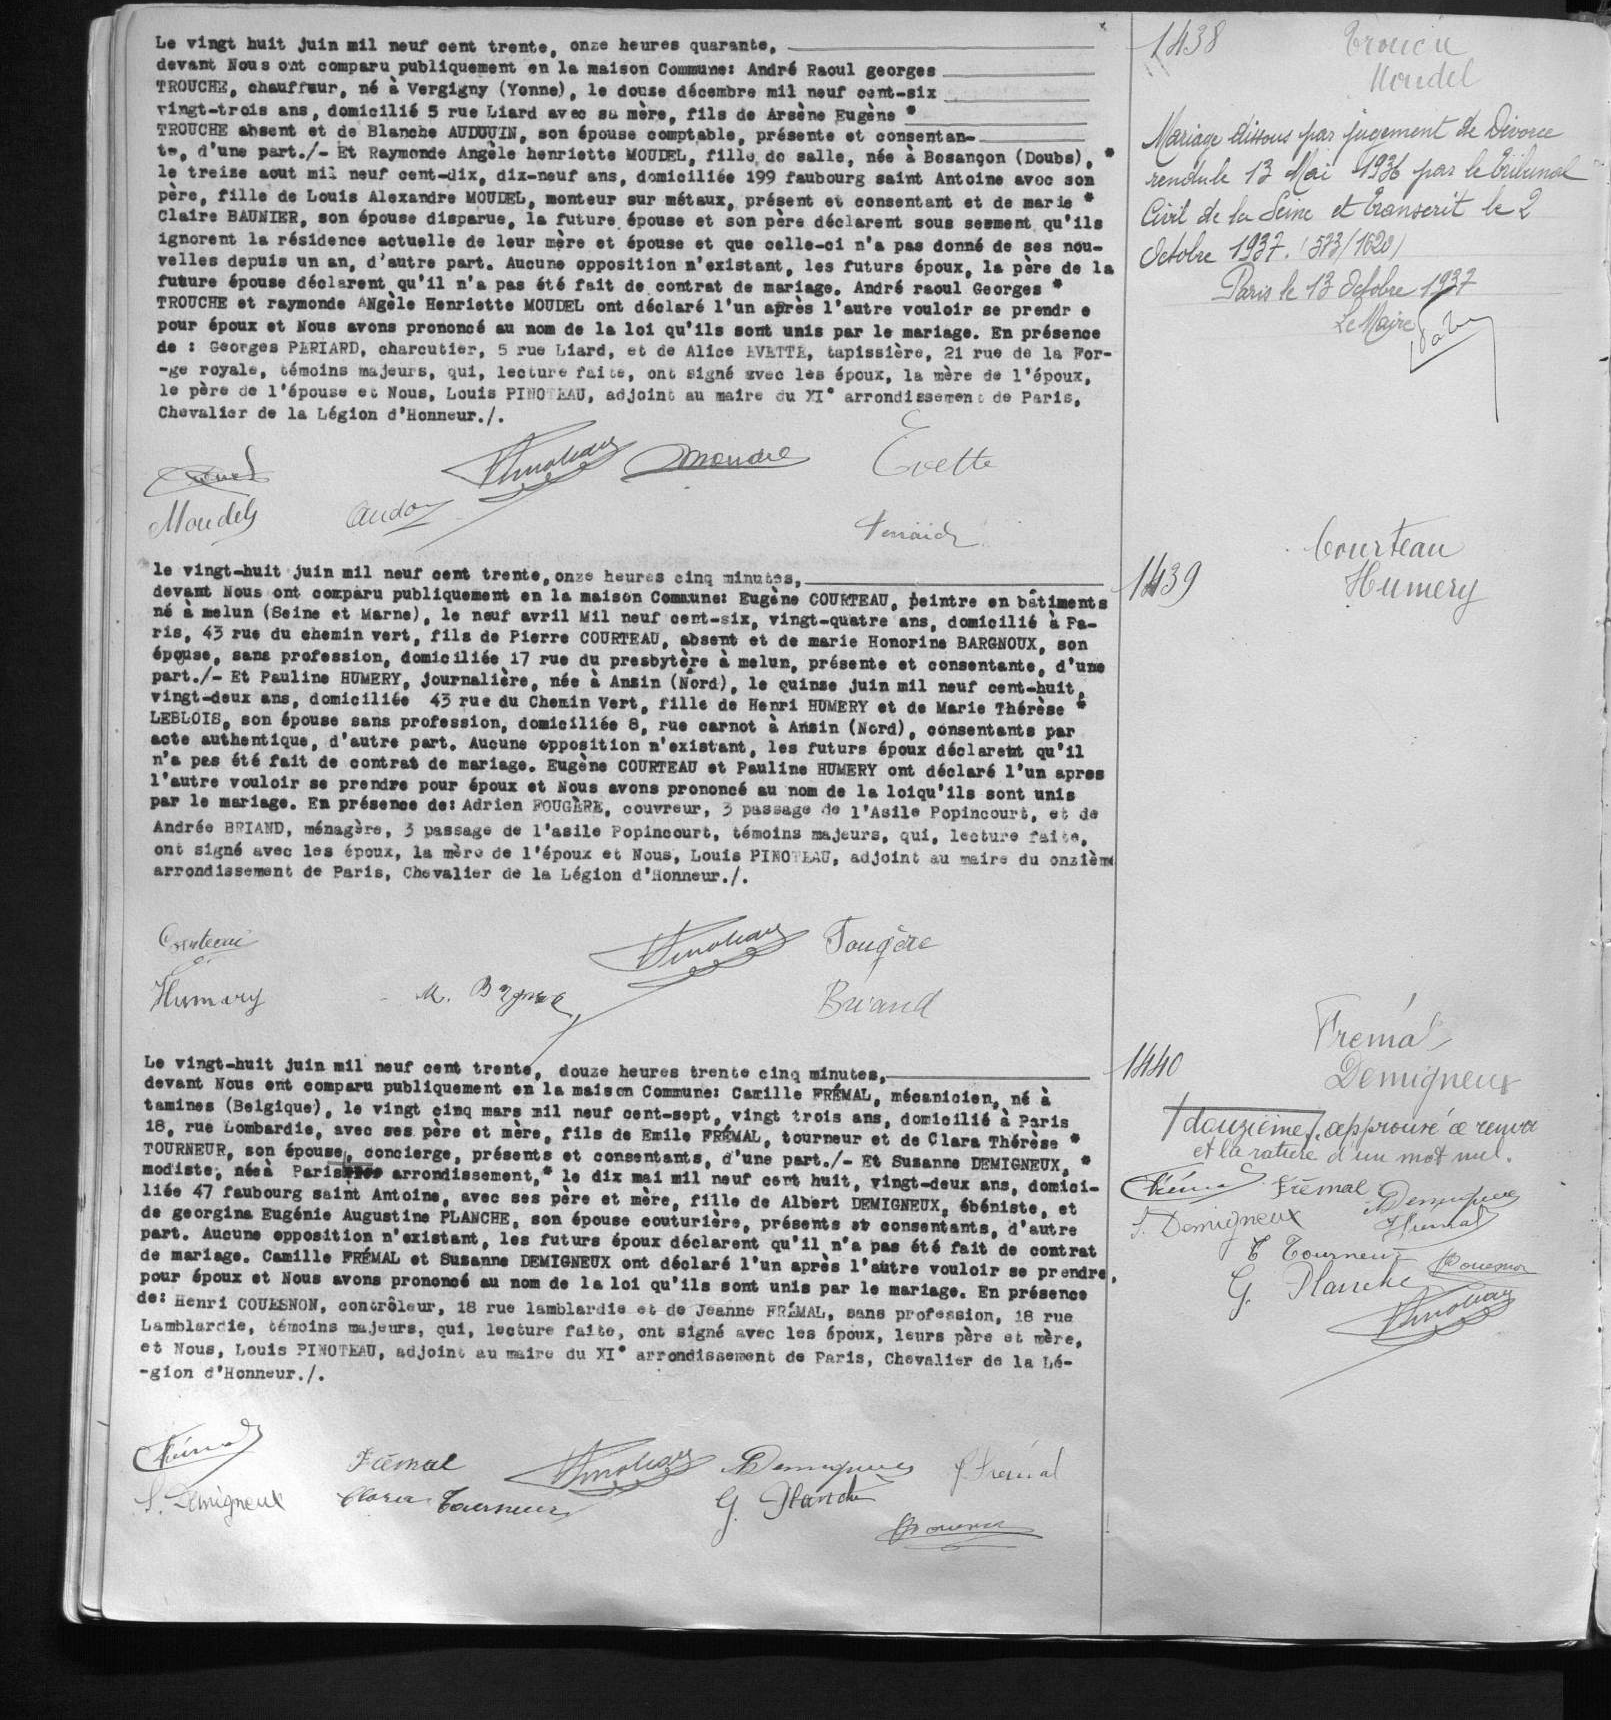Le vingt huit juin mil neuf cent trente, onze heures quarante, -
devant Nous ont comparu publiquement en la maison Commune André Raoul georges -
TROUCHE, chauffeur, né à Vergigny (Yonne), le douze décembre mil neuf cent-six -
vingt-trois ans, domicilié 5 rue Liard avec a mère, fils de Arsène Eugène *-
TROUCH absent et de Blanch ADUUIN, son épouse comptable, présente et consentan -
te, d'une part ./-Et Raymonde Angèle henriette MOUDEL, fille do salle, née à Besançon (Doubs) ,*
le treize aout mil neuf cent-dix, dix-neuf ans, domiciliée 199 faubourg saint Antoine avec son
père, fille de Louis Alexandre MOUDEL, monteur sur métaux, présent et consentant et de marie *
Claire BAUNIER, son épouse disparue, la future épouse et son père déclarent sous serment qu'ils
ignorent la résidence actuelle de leur mère et épouse et que celle-ci n'a pas donné de ses nou-
velles depuis un an, d'autre part. Aucune opposition n'existant, les futurs époux, la père de la
future épouse déclarent qu'il n'a pas été fait de contrat de mariage. André raoul Georges *
TROUCHE et raymonde Angèle Henriette MOUDEL ont déclaré l'un après l'autre vouloir se prendr e
pour époux et Nous avons prononcé au nom de la loi qu'ils sont unis par le mariage. En présence
de: Georges PARIARD, charcutier, 5 rue Liard, et de Alice EVETTE, tapissière, 21 rue de la For-
ge royale, témoins majeurs, qui, lecture faite, ont signé avec les époux, la mère de l'époux,
le père de l'épouse et Nous, Louis PINOTEAU, adjoint au maire du XI ° arrondissemen de Paris,
Chevalier de la légion d'Honneur ./.
le vingt-huit juin mil neuf cent trente, onze heures cinq minutes, -
devant Nous ont comparu publiquement en la maison Commune: Eugène COURTEAU, peintre en bâtiments
né à melun (Seine et Marne), le neuf avril Mil neuf cent-six, vingt-quatre ans, domicilié à Fa-
ris, 43 rue du chemin vert, fils de Pierre COURTEAU, absent et de marie Honorine BARGNOUX, son
épouse, sans profession, domiciliée 17 rue du presbytère à melun, présente et consentante, d'une
part ./-Et Pauline HUMERY, journalière, née à Anzin (Nord), le quinze juin mil neuf cent-huit,
vingt-deux ans, domiciliée 43 rue du Chemin Vert, fille de Henri HUMERY et de Marie Thérèse *
LEBLOIS, son épouse sans profession, domiciliée 8, rue carnot à Ansin (Nord), consentants par
acte authentique, d'autre part. Aucune opposition n'existant, les futurs époux déclarent qu'il
n'a pas été fait de contrat de mariage. Eugène COURTEAU et Pauline HUMERY ont déclaré l'un apres
l'autre vouloir se prendre pour époux et Nous avons prononcé au nom de la loi qu'ils sont unis
par le mariage. En présence de: Adrien FOUGERE, couvreur, 3 passage de l'Asile Popincourt, et de
Andrée BRIAND, ménagère, 3 passage de l'asile Popincourt, témoins majeurs, qui, lecture faite,
ont signé avec les époux, la mère de l'époux et Nous, Louis PINOTEAU, adjoint au maire du onzième
arrondissement de Paris, Chevalier de la Légion d'Honneur ./.
Le vingt-huit juin mil neuf cent trente, douze heures trente cinq minutes -
devant Nous ont comparu publiquement en la maison Commune: Camille FREMAL, mécanicien, né à
tamines (Belgique), le vingt cinq mars mil neuf cent-sept, vingt trois ans, domicilié à Paris
18, rue Lombardie, avec ses père et mère, fils de Emile FREMAL, tourneur et de Clara Thérèse *
TOURNEUR, son épouse, concierge, présents et consentants, d'une part ./-Et Suzanne DEMIGNEUX ,*
modite, née à Paris arrondissement, * le dix mai mil neuf cent huit, vingt-deux ans, domici-
liée 47 faubourg saint Antoine, avec ses père et mère, fille de Albert DEMIGNEUX, ébéniste, et
de georgina Eugénie Augustine PLANCHE, son épouse couturière, présents et consentants, d'autre
part. Aucune opposition n'existant, les futurs époux déclarent qu'il n'a pas été fait de contrat
de mariage. Camille FREMAL et Suzanne DEMIGNEUX ont déclaré l'un après l'autre vouloir se prendre
pour époux et Nous avons prononcé au nom de la loi qu'ils sont unis par le mariage. En présence
de: Henri COUESNON, contrôleur, 18 rue lamblardie et de Jeanne FREMAL, sans profession, 18 rue
Lamblardie, témoins majeurs, qui, lecture faite, ont signé avec les époux, leurs père et mère,
et Nous, Louis PINOTEAU, adjoint au maire du XI ° arrondissement de Paris, Chevalier de la Lé-
gion d'Honneur ./.
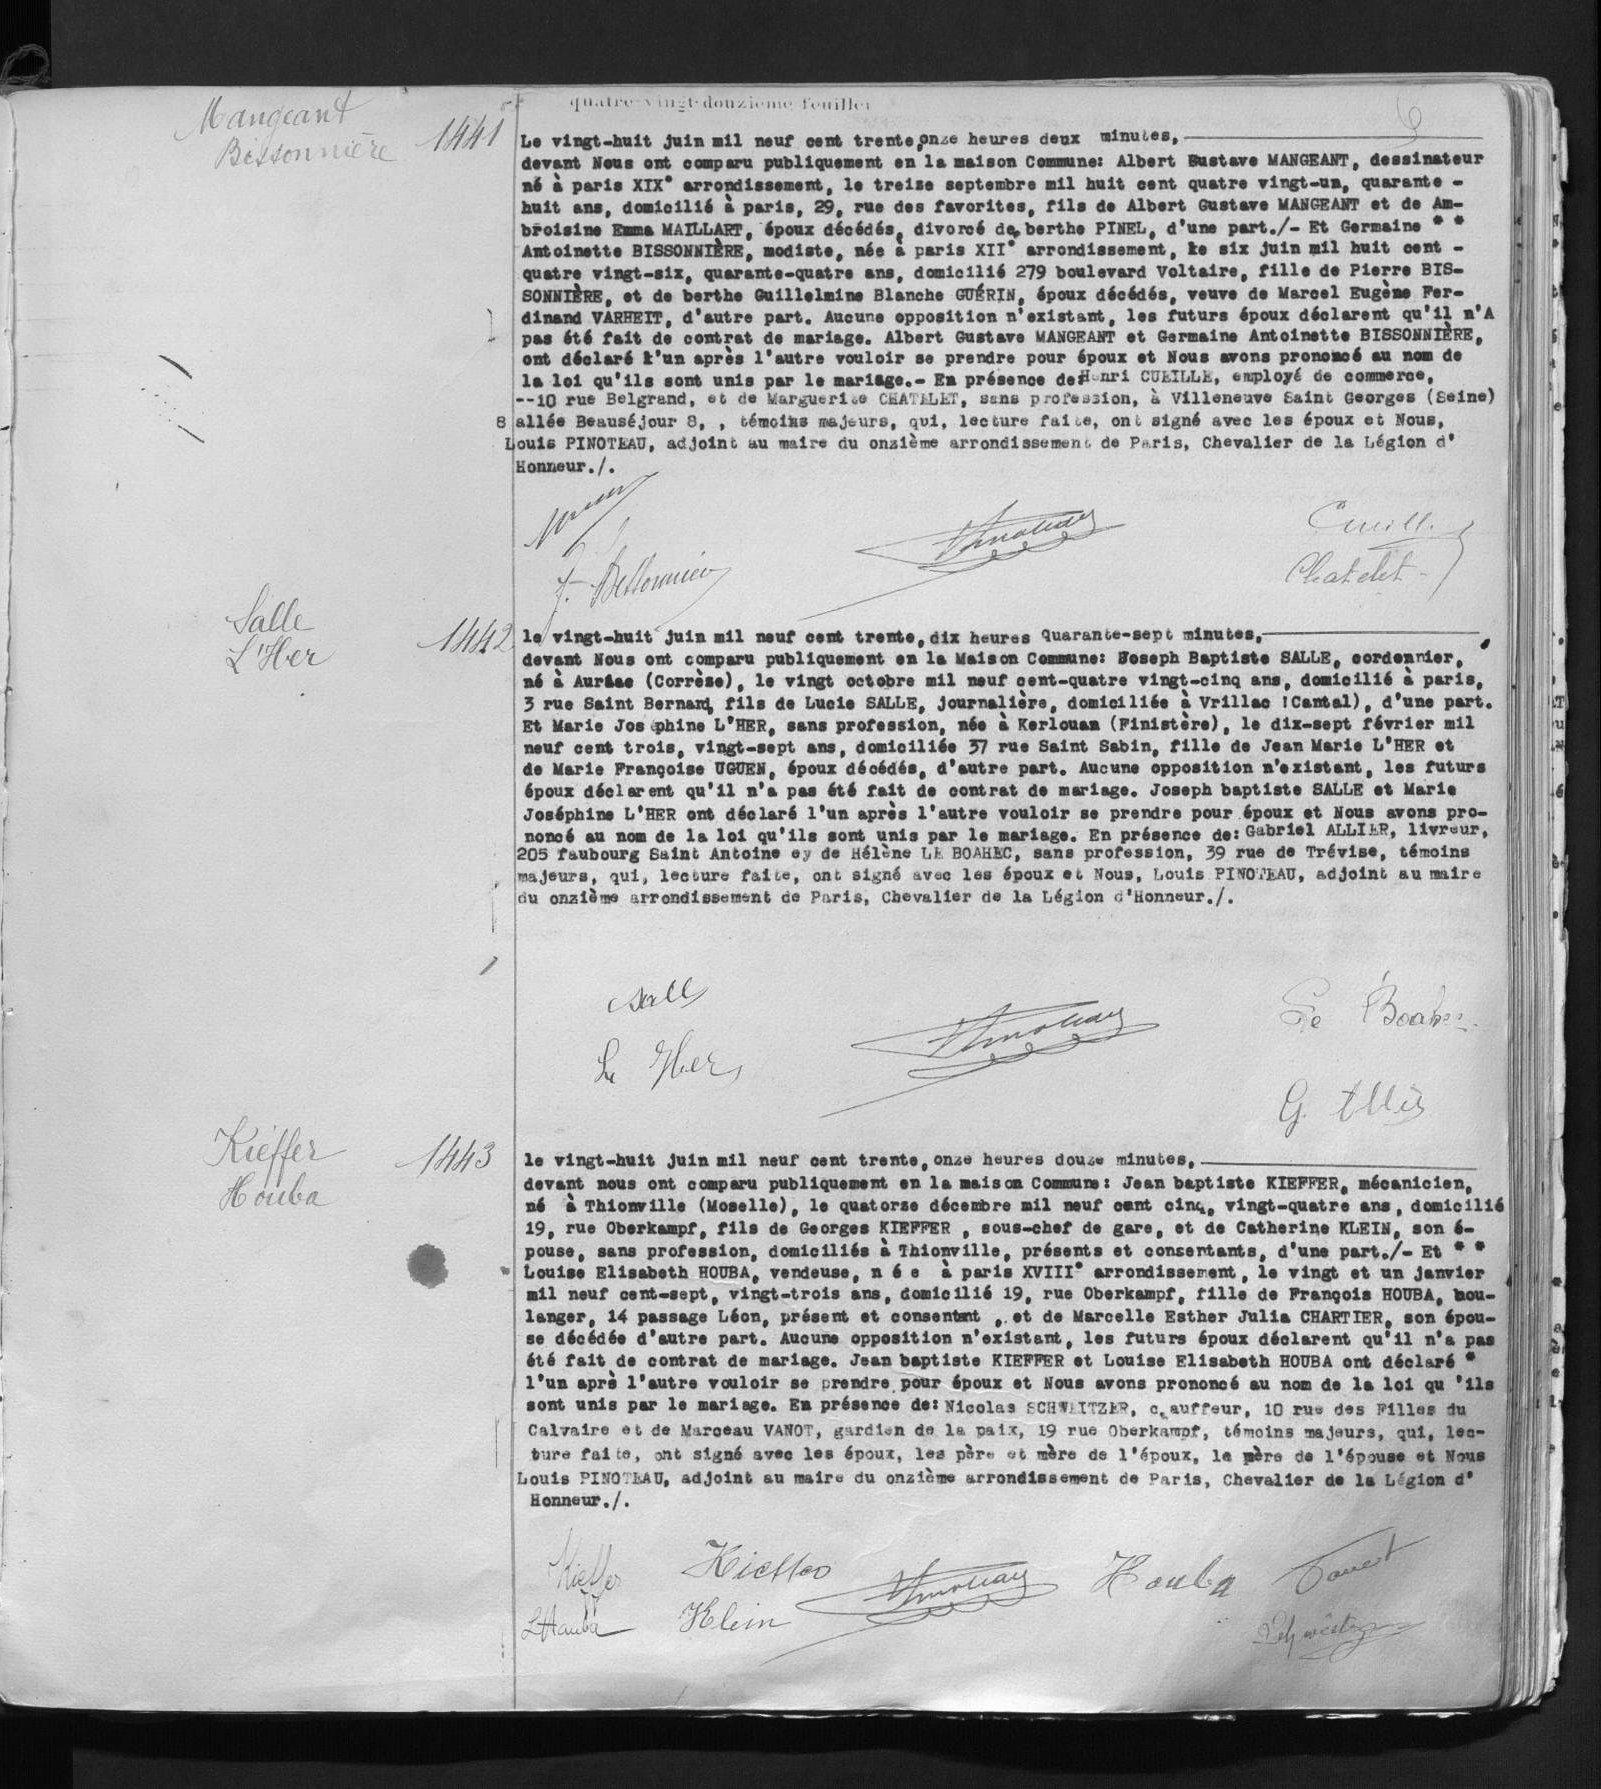Le vingt-huit juin mil neuf cent trente, onze heures deux minutes, -
devant Nous ont comparu publiquement en la maison Commune: Albert Eustave MANDEANT, dessinateur
né à paris XIX ° arrondissement, le treize septembre mil huit cent quatre vingt-un, quarante-
huit ans, domicilié à paris, 29, rue des favorites, fils de Albert Gustave MANGEANT et de Am-
broisine Emma MAILLART, époux décédés, divorcé de berthe PINEL, d'une part ./-Et Germaine **
Antoinette BISSONNIERE, modiste, née à paris XII ° arrondissement, le six juin mil huit cent-
quatre vingt-six, quarante-quatre ans, domicilié 279 boulevard Voltaire, fille de Pierre BIS-
SONNIERE, et de berthe Guillelmine Blanche GUERIN, époux décédés, veuve de Marcel Eugène Fer-
dinand VARHEIT, d'autre part. Aucune opposition n'existant, le futurs époux déclarent qu'il n'A
pas été fait de contrat de mariage. Albert Gustave MANGEANT et Germaine Antoinette BISSONIERE,
ont déclaré l'un après l'uatre vouloir se prendre pour époux et Nous avons prononcé au nom de
la loi u'ils sont unis par le mariage .-Et présence de Henri CUEILLE, employé de commerce,
-10 rue Belgrand, et de Marguerite CHATELET, sans profession, à Villeneuve Saint Georges (Seins)
allée Beauséjour 8, témoins majeurs, qui, lecture faite, ont signé avec les époux et Nous,
Louis PINOTEAU, adjoint au maire du onzième arrondissement de Paris, Chevalier de la Légion d'
Honneur ./.
Le vingt-huit juin mil neuf cent trente, dix heures quarante-sept minutes, -,
devant Nous ont comparu publiquement en la Maison Commune: Joseph Baptiste SALLE, cordonnier,
né à Aurâce (Corrèze), le vingt octobre mil neuf cent-quatre vingt-cinq ans, domicilié à paris,
3 rue Saint Bernard, fils de Lucie SALLE, journalière, domiciliée à Vrillac: Cantal), d'une part.
Et Marie Joséphine L'HER, sans profession, née à Kerlouan (Finistère), le dix-sept février mil
neuf cent trois, vingt-sept ans, domiciliée 37 rue Saint Sabin, fille de Jean Marie L'HER et
de Marie Françoise UGUEN, époux décédés, d'autre part. Aucune opposition n'existant, es futurs
époux déclarent qu'il n'a pas été fait de contrat de mariage. Joseph baptiste SALLE et Marie
Joséphine L'HER ont déclaré l'un après l'autre vouloir se prendre pour époux et Nous avons pro-
noncé au nom de la loi qu'ils sont unis par le mariage. En présence de: Gabriel ALLIER, livreur,
205 faubourg Saint Antoine ey de Hélène LE BOAHEC, sans profession, 39 rue de Trévise, témoins
majeurs, qui, lecture faite, ont signé avec les époux et Nous, Louis PINOTEAU, adjoint au maire
du onzième arrondissement de Paris, Chevalier de la Légion d'Honneur ./.
Le vingt-huit juin mil neuf cent trente, onze heures douze minutes, -
devant nous ont comparu publiquement en la maison Commune: Jean baptiste KIEFFER, mécanicien,
né à Thionville (Moselle), le quatorze décembre mil neuf cent cinq, vingt-quatre ans, domicilié
19, rue Oberkampf, fils de Georges KIEFFER, sous-chef de gare, et de Catherine KLEIN, son é-
pouse, sans profession, domiciliés à Thionville, présents et consentants, d'une part ./-Et **
Louise Elisabeth HOUBA, vendeuse, n é e à paris XVIII arrondissement, le vingt et un janvier
mil neuf cent-sept, vingt-trois ans, domiclié 19, rue Oberkampf, fille de François HOUBA, hou-
langer, 14 passage Léon, présent et consentant, et de Marcelle Esther Julia CHARTIER, son épou-
se décédée d'autre part. Aucune opposition n'existant, les futurs époux déclarent qu'il n'a pas
été fait de contrat de mariage. Jean baptiste KIEFFER et Louis Elisabeth HOUBA ont déclaré *
l'un après l'autre vouloir se prendre pour époux et Nous avons prononcé au nom de la loi qu'ils
sont unis par le mariage. En présence de: Nicolas SHCHWIETZER, chauffeur, 10 rue des filles du
Calvaire et de Marceau VANOT, gardien de la paix, 19 rue Oberkampf, témoins majeurs, qui, lec-
ture faite, ont signé avec les époux, les père et mère de l'époux, la mère de l'épouse et Nous
Louis PINOTEAU, adjoint au maire du onzième arrondissement de Paris, Chevalier de la Légion d'
Honneur ./.